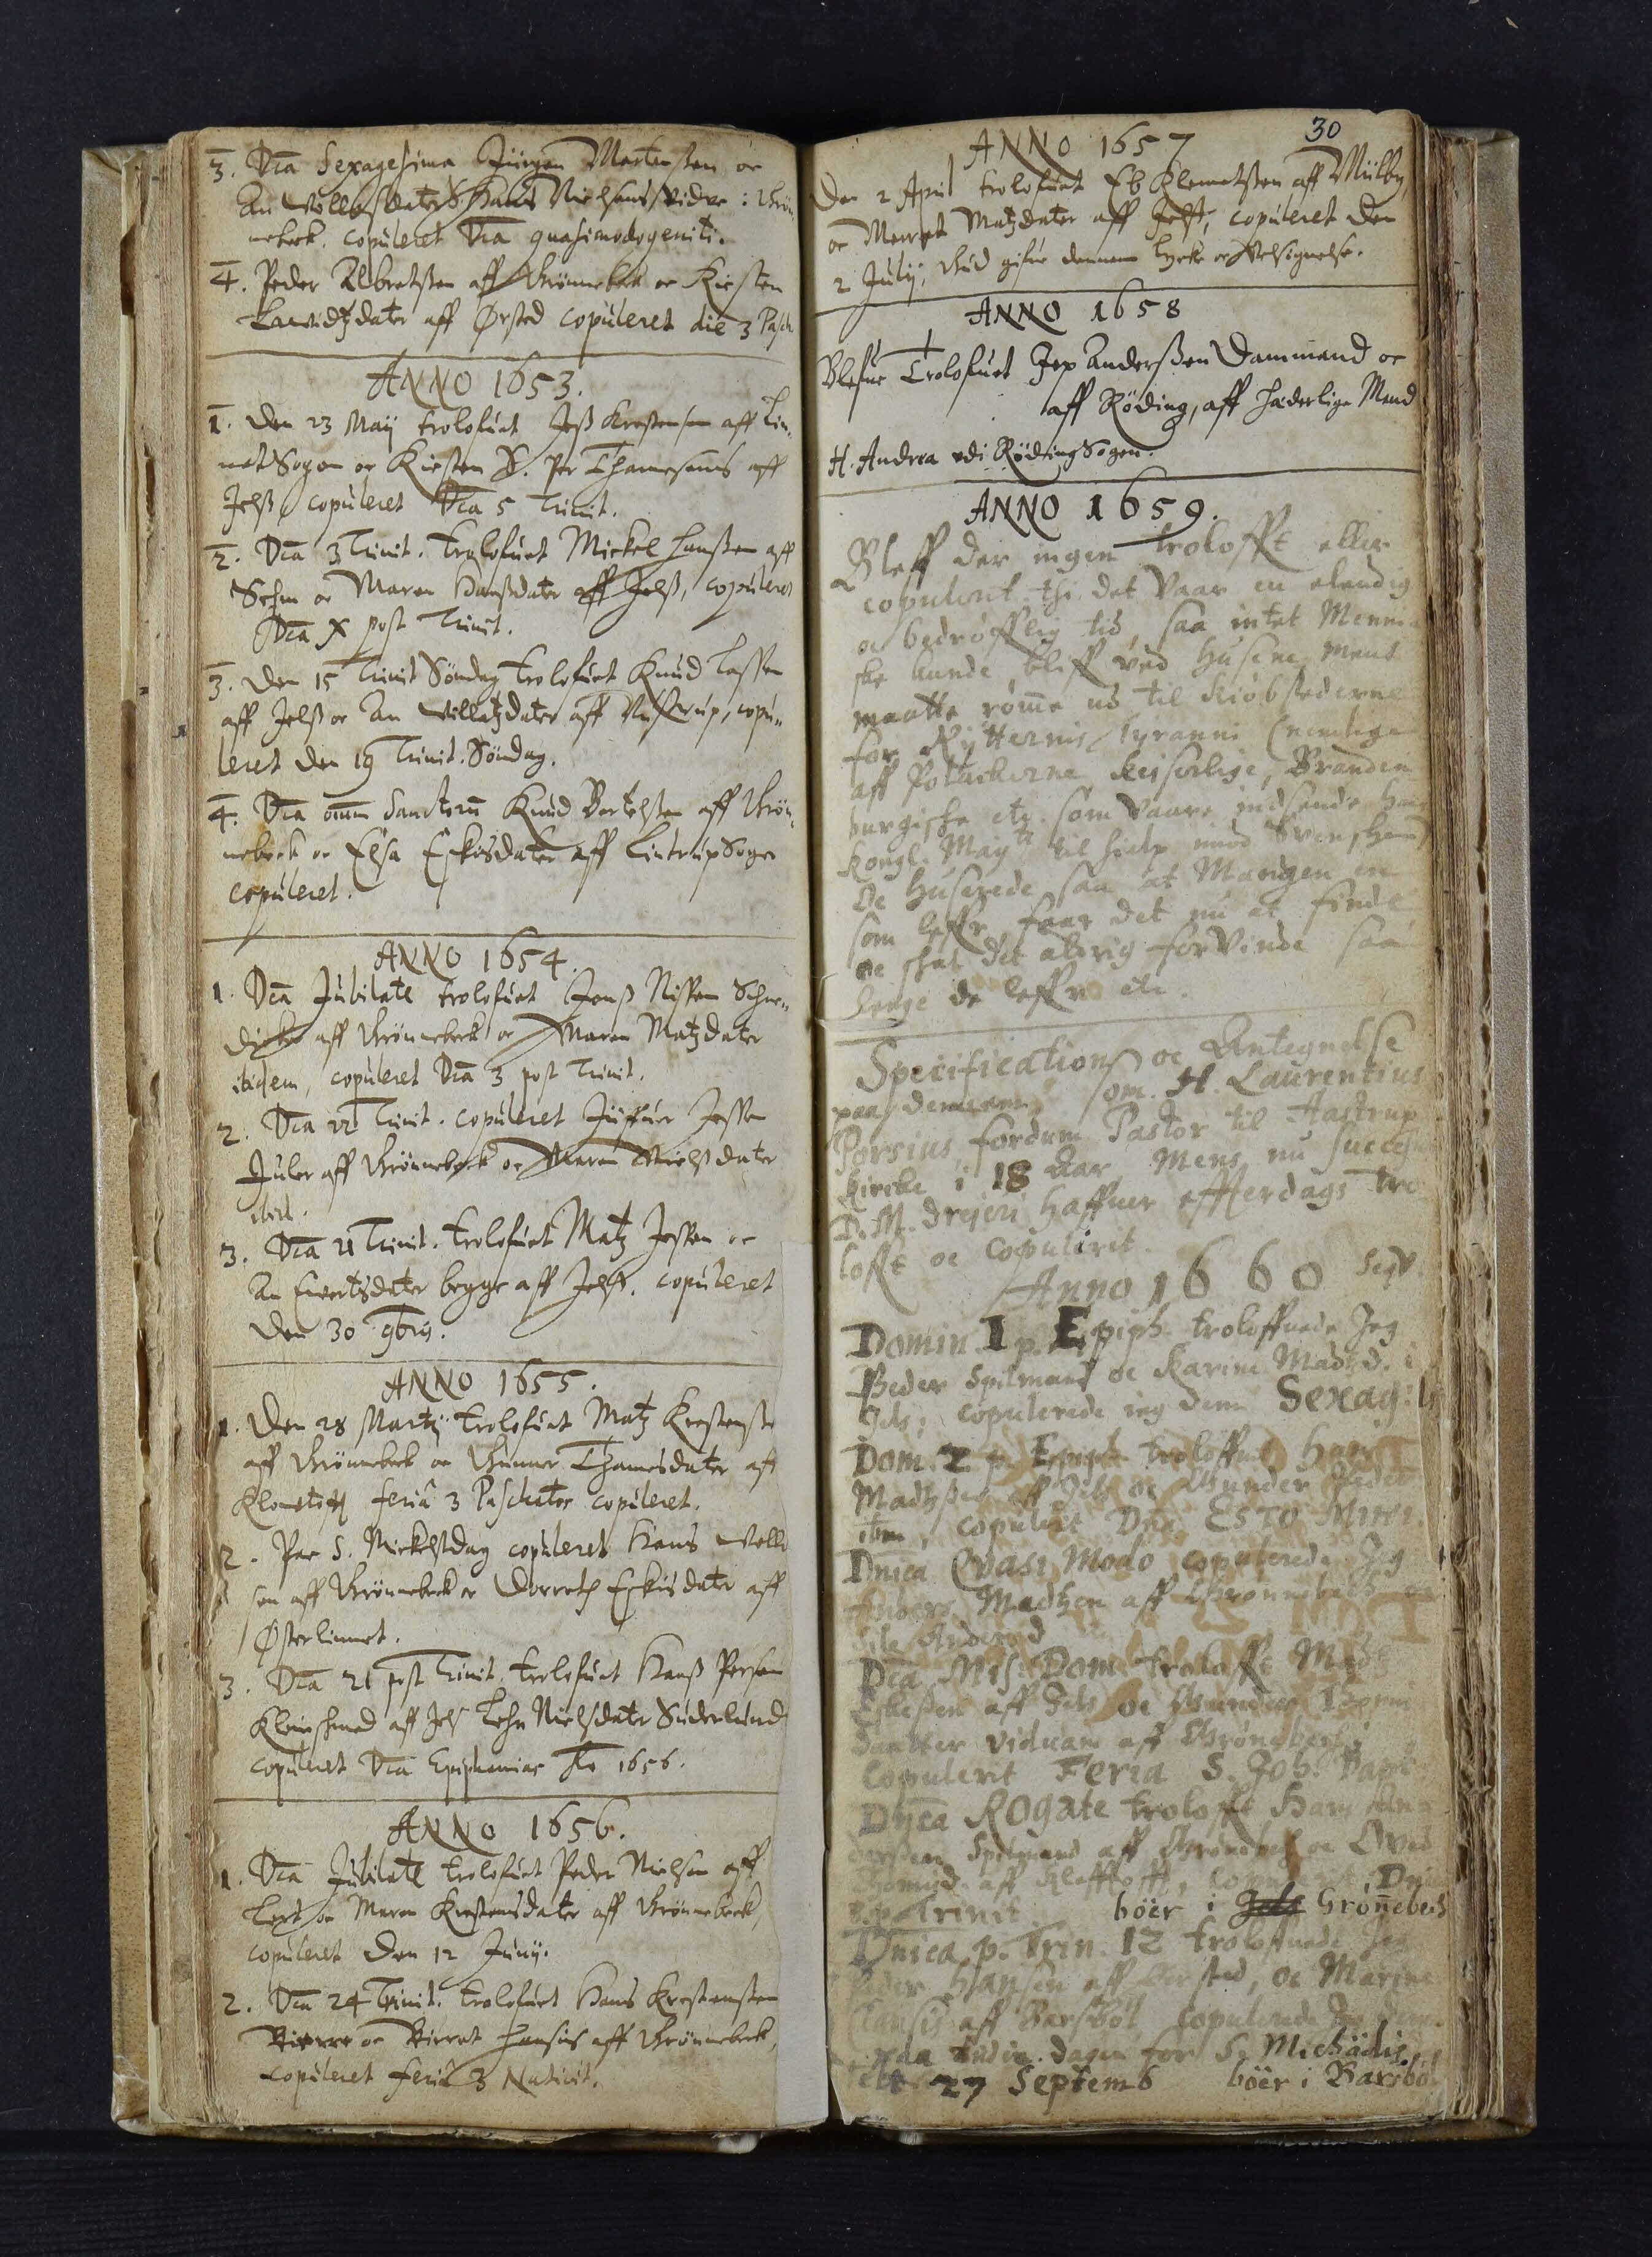3. Dca Sexagesima Jurgen Martensen oc
An Vollesdater S. Hans Nielsens Vida## i Grøn
nebeck copuleret Dca quasimodogemiti.
4. Peder Albretssen aff Grønnebeck oc Kiesten
Lavidtzdater aff Ørsted copuleret die 3 Pasch
ANNO 1653.
1. Den 23 Maij trolofuet Jess Krestensen aff Lin¬
net Sogen oc Kiesten S. Per Thamesens aff
Jelss copuleret Dca 5 Trinit.
2. Dca 3 Trinit. Trolofuet Mickel Hanssen aff
Sehm oc Maren Hanssdater aff Jelss, copuleret
Dca x post Trinit.
3. Den 15 Trinit Søndag trolofuet Knud Lassen
aff Jelss oc An Villatzdater aff Nustrup, copu¬
leret den 19 Trinit. Søndag.
4. Dca Otum Santoru Knud Bertelssen aff Grøn¬
nebeck oc Elsa Eskisdater aff Lintrup Sogn
copuleret.
ANNO 1654.
1. Dca Jubilate trolofuet Jenss Nissen Schne¬
dicker aff Grønnebeck oc Maren Matzdater
ibidem, copuleret Dca 3 post Trinit.
2. Dca 22 Trinit. Copuleret Juffue Jessen
Juler aff Grønnebeck oc Maren Nielssdater
ibid.
3. Dca 21 Trinit. Trolofuet Matz Jessen oc
An Evertsdater begge aff Jelss, copuleret
den 30 9tris.
ANNO 1655.
1. Den 28 Martij trolofuet Matz Krestenssen
aff Grønnebeck oc Gunner Thamesdater aff
Klovtoft Feria 3 Paschator copuleret.
2. Paa S. Mickelsdag copuleret Hans Vol##
sen af Grønnebeck oc Dorreth Eskisdater aff
Østerlinnet.
3. Dca 21 post Trinit trolofuet Hanss Persen
Kleinschmed aff Jels Lehn Nielsdater Suderlund
copuleret Dca Epiphemias An 1656.
ANNO 1656.
1. Dca Jubilate trolofuet Peder Nielsen aff
Lert oc Maren Krestensdater aff Grønnebeck,
copuleret Den 12 Junij.
2. Dca 24 Trini. trolofuet Hans Krestenssen
oc Birret Hansis aff Grønnebeck
copuleret Feria 3 Nativit.
30
ANNO 1657
Den 2 April trolofuet Eb Klemetssen aff Mulby
oc Merret Matzdater aff Jelss, copuleret den
2 Julij, Gud gifue dennem Lycke oc Velsignelse.
Anno 1658
Blefue Trolofuet Jep Anderssen Dammand oc
aff Røding, aff hæderlige
H. Andera vdi Røding Sogen.
ANNO 1659.
Bleff der ingen troloffe eller
copuleret thi det vaar en elendig
oc bedrøfflig tid, saa intet Menni
ske kunde bliff ved husene mens
maatte rømme ud til Kiøbstederne
for Rytternis Tyranni (nemlige
aff Polackerne keiserlige, Branden
burgiske etc. som vaare indsende Hans
Kongl. May ts til hielp mod Svensken)
De huserede saa at Mangen en
som leffe, faar det nu at finde
oc skal det aldrig forvinde saa 
lenge de leffe etc.
Specification oc Antegnelse
paa dennem som H. Laurentius 
Porsius fordum Pastor til Aastrup 
Kircke i 18 Aar, mens nu
D. M. Drejeri haffuer effterdags tro
lofft oc copuliret.
Segv##
Anno 1660
Domin I p. Epiph troloffuede Jeg
Peder Spilmand oc Karine Madtzd. i
Jels; copulerede ieg dem Sexag:
Dom 2 p. Epiph. Troloffuet Hans
Madtssen aff Jels oc Gunder Peders
ibm, copuleret Dca ESTO MIHI.
Dnica Qvasi Modo copulerede. Jeg
Anders Madtsen aff Grønnebeck oc
Sile Andersd
Dca Mis Dom trolofte Madtz 
Eskessen aff Jels oc Gunder Thomis
Daatter viduam aff Grønebech.
Copulerit Feria S. Joh. Bapt
Dnca Rogate trolofte Hans An¬
dersen Spilmand aff Grønebech oc Oved
Thomisd. aff Kloftofft, copulerit i
8 p Trinit. Boer i Jels Grønebech
Dnica p. Trini 12 trolifuede Jeg
Peder Hansen aff Ørsted, oc Marine 
Clausis aff Barsbøl, copulerede Jeg Dem 
paa t##d## Dagen for S. Michaelis.
27 Septemb boer i Barsbøl